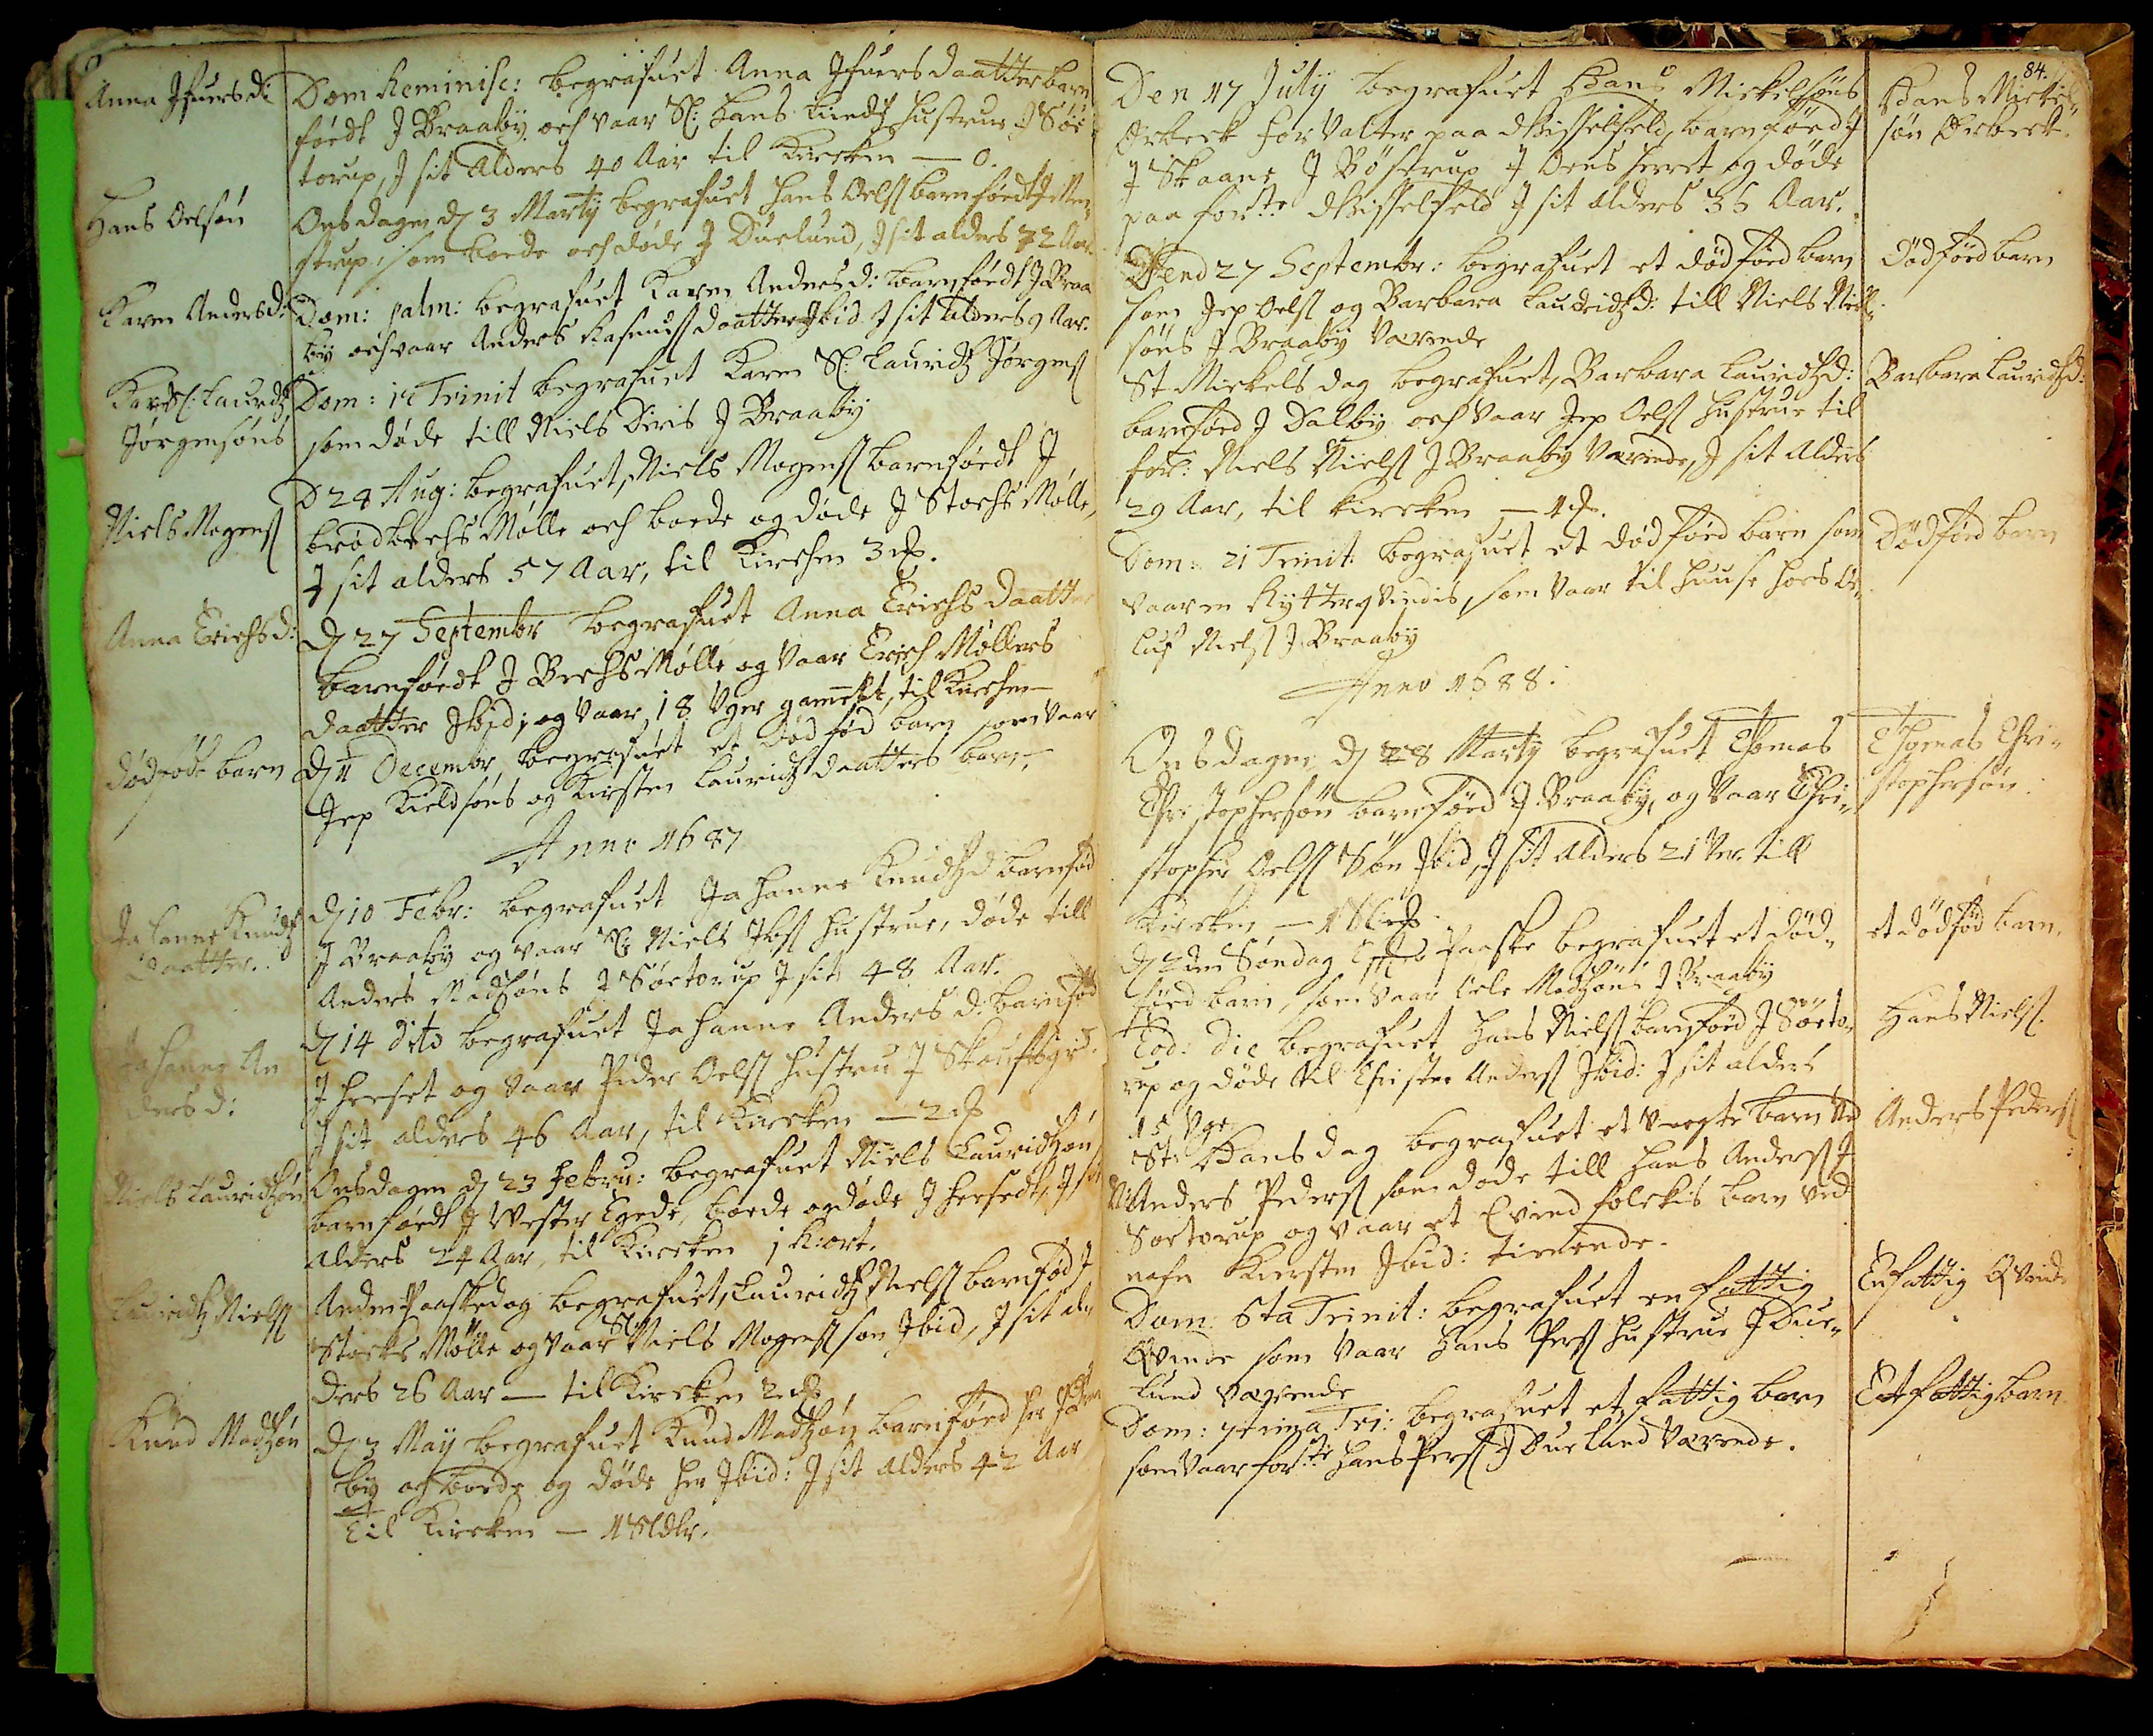Anna Jfuersd:
Dom Reminise: begrafuet Anna Jfuersdaatter barn
 føedt J Braaby och Vaar Sl: Hans Knuds## Hustrue J Søe
torup, J sit Alders 40 Aar til Kircken -0.
Hans Oelsøn
¬Onsdagen d 3. Marty begrafuet Hans Oels barnføedt J Men¬
strup, som boede och døde J Duelund, J sit alders 72 Aar.
Karen Andersd:
Dom: Palm: begrafuet Karen AndersD: barnføedt J Braa
by och vaar Anders Rasmus daatter Jbid, J sit alders 69 Aar.
Karen SL Lauridz##
Jørgensøns
Dom: 14 Trinit begrafuet Karen Sl: Lauridz Jørgens
som døde till Niels Diris J Braaby
Niels Mogens
D. 28 Aug: begrafuet, Niels Mogens barnføedt J
Brødbechs Mølle och boede og døde J Stochs Mølle,
J sit alders 57 Aar, til Kirchen 3 Mk.
Anna Erichsd:
D. 27 Septembr begrafuet Anna Erichsdaatter
barnføedt J Bechs Mølle og Vaar Erich Møllers
daatter Jbid; og Vaar 18 Uger gammelt, til Kirchen -
Dødfødt Barn 
D. 1 Decembr; begrafuet et dødfød barn som Vaar
Jep Kieldsøns og Kirsten Lauridzdaatters barn -
Anno 1687
Johanne Knuds
daatter 
D. 10 Febr: begrafuet Johanne Knudsd. barnføedt
J Braaby og Vaar Sl : Niels Jbs Hustrue, døde till
Anders Madsøns J Søetorup J sit 48 Aar.
Johanne An
dersd:. 
D. 14. Dito begrafuet Johanne Andersd. barnføedt
J Herset og vaar Peder Oels hustrue J Skoufsgrd##,
J sit alders 46 Aar, til Kircken - 2 Mk
Niels Lauridzøn
Onsdagen d 23 Febru: begrafuet Niels Lauridzøn
barnføedt J Vester Egede, boede och døde J Hersedt, J sit
alders 24 Aar, til Kircken 1 R: ort##
Lauridz Niels
Anden Paaskedag begrafuet Lauridz Niels, barnføed J
Stocks Mølle og Vaar SL Niels Mogens  Søn Jbid, J sit al¬
ders 26 Aar - til Kircken 2 Mk.##
Knud Madzøn
D. 3 May begrafuet Knud Madzøn, barnføed her J Braa
by## og boede og døde her Jbid: J sit alders 42 Aar.
Til Kircken - 1 Sl. dlr.
Dend 17 July begrafuet Hans Mickelsøns
Gebert## forvalter paa Gisselfeld, barnføed J
J Skaane J Bøstrup i Ornsherret og døde
paa forte Gisselfeld J sit alders 36 Aar.
84.
Hans Mickel
søn Gebert##
Dend 27 Septembr: begrafuet et dødføed Barn
som Jep Oels og Barbara Lauridzd: till Niels Niel¬
søns J Braaby Værende. 
Dødføed barn
Sk. Mickels dag begrafuet, Barbara Lauridsd:
barnføed J Dalby och Vaar Jep Oels hustrue til
fort## Niels Niels J Braaby Værende, J sit aldris
29 Aar, til Kircken - 1 Mk.
Barbara Lauridsd:
Dom: 21 Trinit. begrafuet et dødføed barn som
vaar en Rytterqvindis som Vaar til huuse hoes Oe¬
luf Niels J Braaby.
Dødføed barn
Anno 1688:
Onsdagen d 28 Marty begrafuet Thomas
Christophersøn barnføed J Braaby, og Vaar Chri¬
stopher Oels Søn Jbid, J sit Alders 21 Uer##, till
Kircken - 1 Sl Mark. 
Thomas Chri¬
stophersøn
D. 2dn Søndag Efter Paaske begrafuet et død
føed barn, som Vaar Oele Madz J Braaby.
Et dødfød barn
Eod: die begrafuet Hans Niels barnføed J Søeto¬
rup og døde til Christen Anders Jbid: J sit aldris
15 Uge. 
Hans Niels
St Hans dag begrafuet et U cgte Barn ##
## Anders Peders, som døde till Hans Anders J
Søetorup og Vaar et Qvindfolkis barn ved
Nafn Kirsten Jbid: tienende.
Anders Peders
Dom 6ta Trinit: begrafuet en fattig
Qvinde som Vaar Hans Pers hustrue, J Due¬
lund Værende.
En fattig Qvinde
Dom: 7tima Tri: begrafuet et fattig barn
som vaar forte## Hans Pers J Duelund Værende.
Eet fattig barn
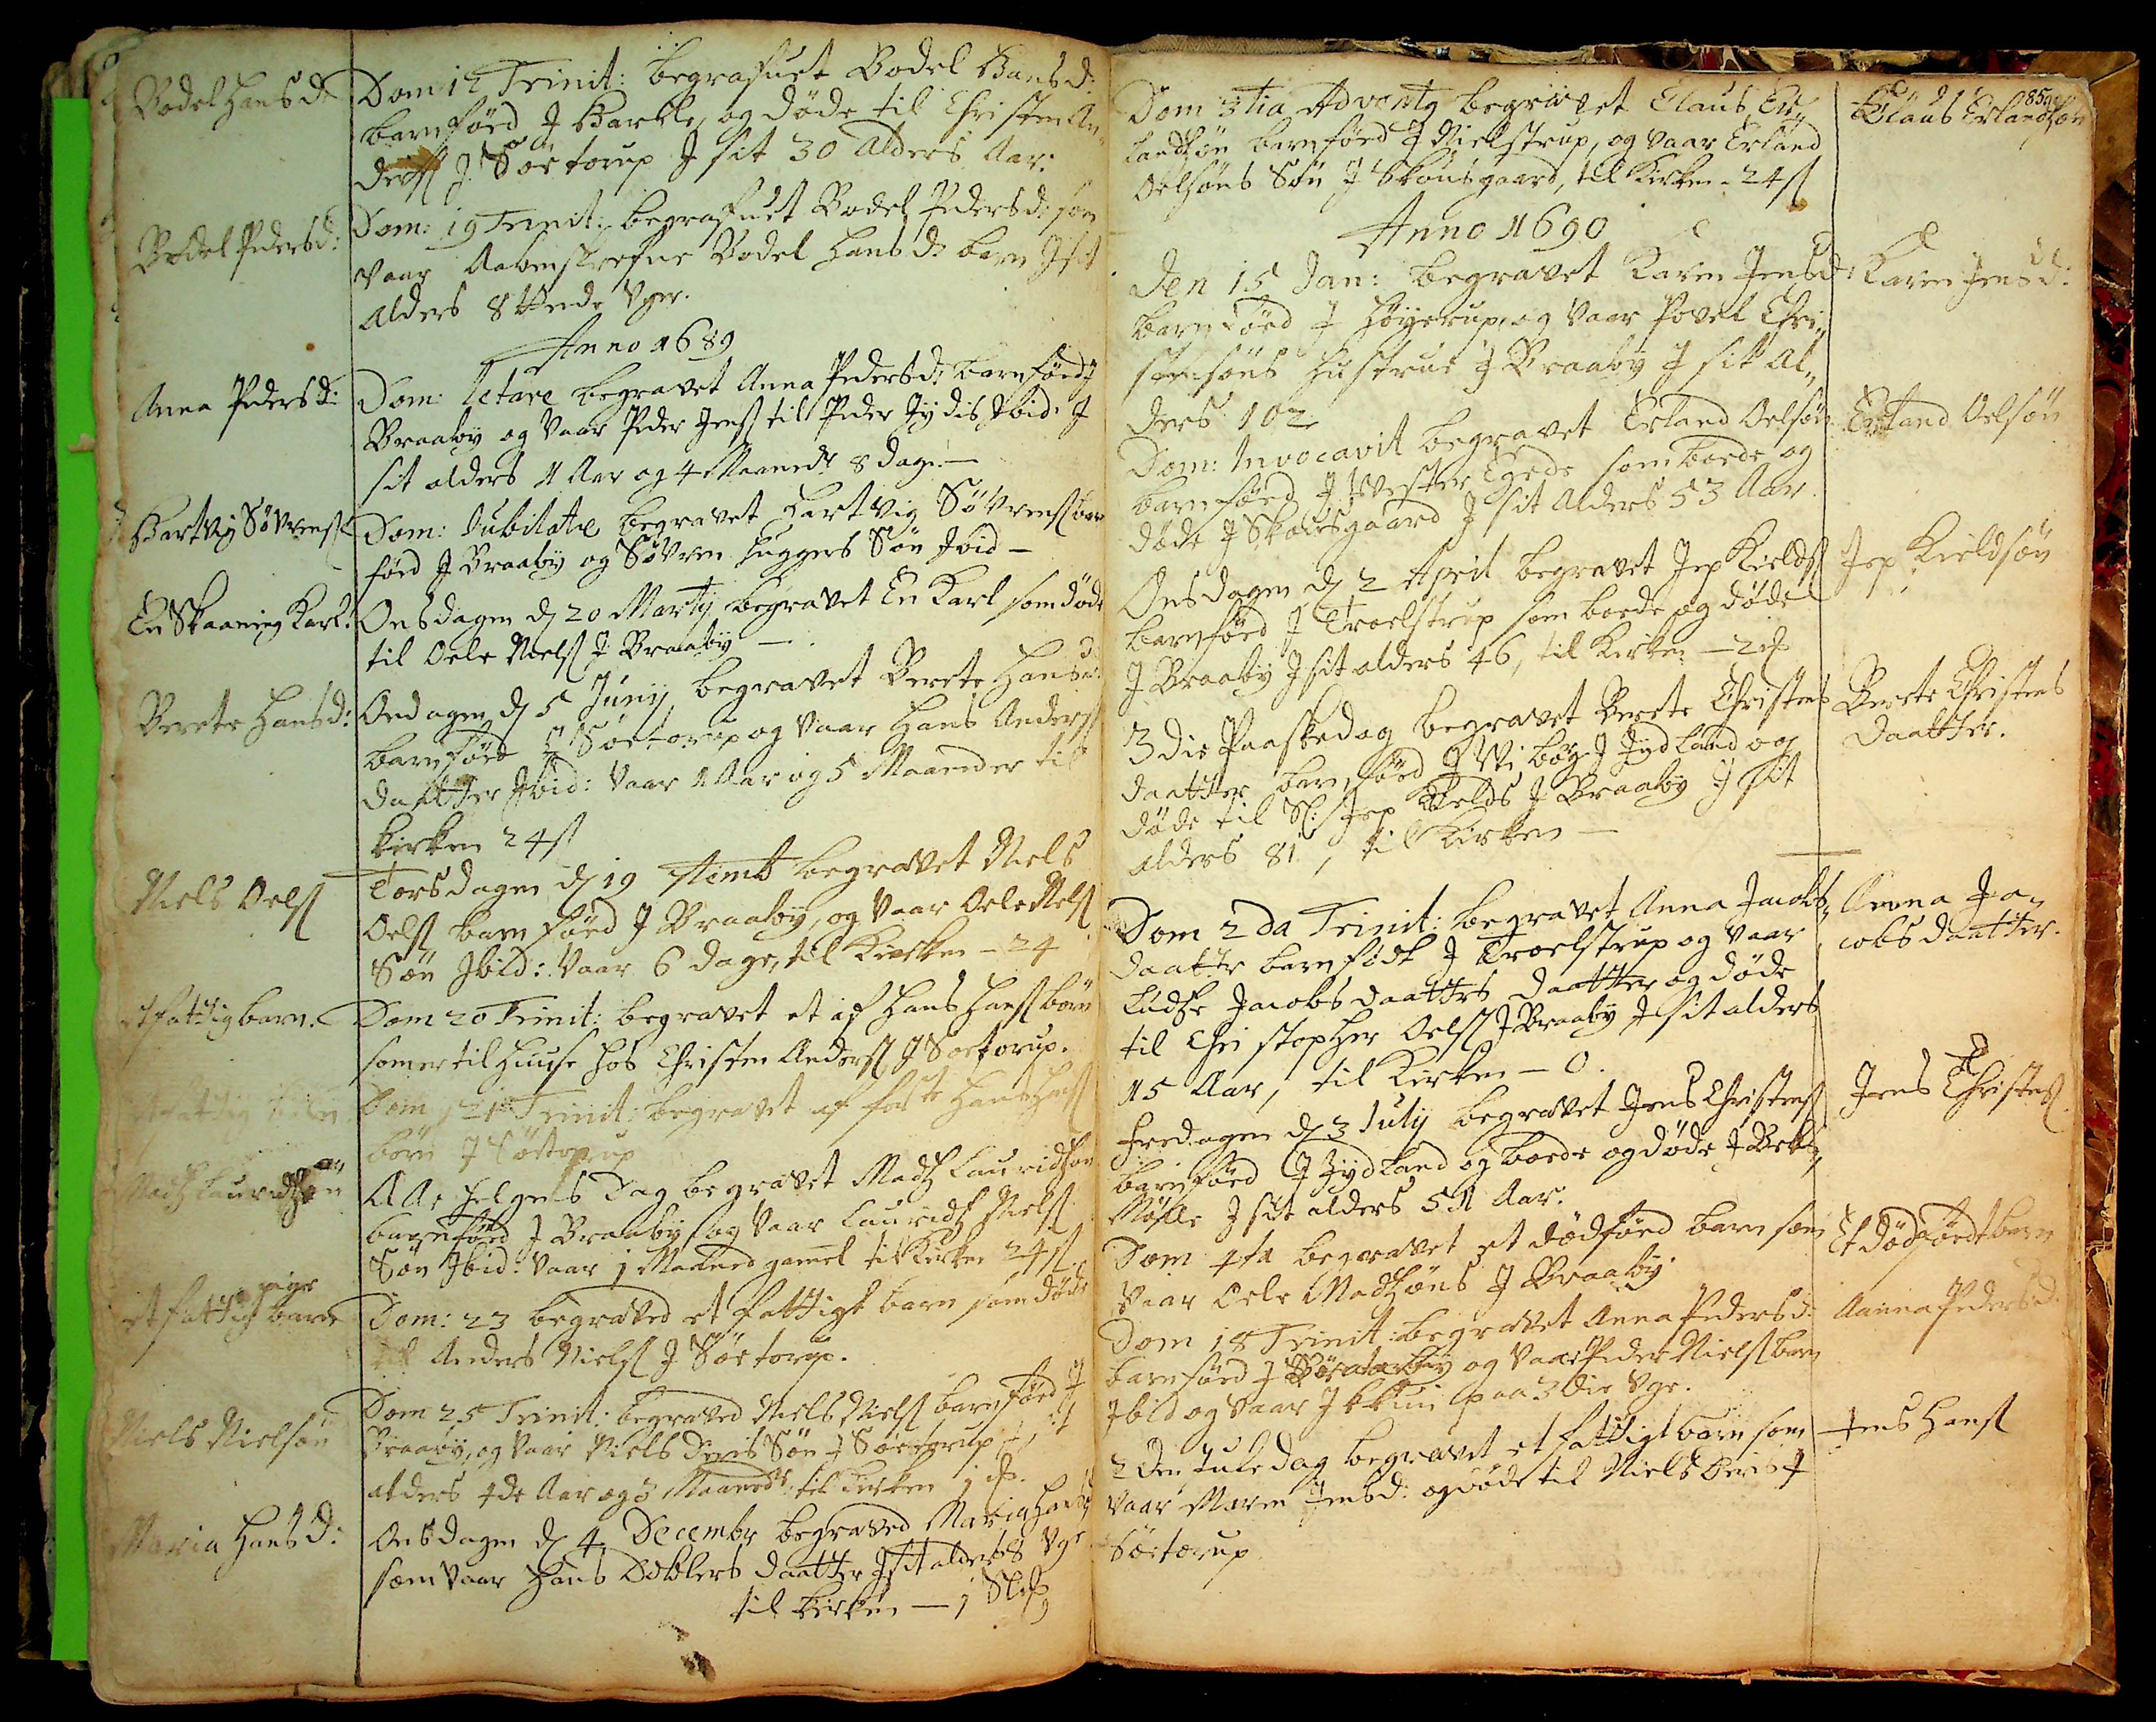Bodil Hansd: 
Dom: 12 Trinit: begrafuet Bodil Hansd:
barnføed J Harsle, og døde til Christen An¬
dersøn J Søetorup i sit 30 Alders Aar.
Bodel Pedersd:
Dom: 19 Trinit: Begrafuet Bodel Pedersd: som
Vaar Aabmskrifne Bodel Hansd: Barn, J sit
alders 8ttende## Uger.
Anno 1689
Anna Pedersd:
Dom: Letare begravet Anna Pedersd: barnføed J
Braaby og vaar Peder Jens til Peder Jydis Jbid, J
sit alders 1 Aar og 4 Maaned 8 Dage -
Hartvig Søvrensøn
Dom: Jubilate begravet Hartvig Søvrensøn barn
føed J Braaby og Søvren Huggers Søn Jbid -
En Skaaning Karl
Onsdagen d. 20 Marty begravet En Karl som døde
til Oele Niels J Braaby. -
Berete Hansd: 
Onsdagen d 5 Juny begravet Berete Hansd.
barnføed J Søetorup og Vaar Hans Anders
daatter Jbid: Vaar 1 Aar og 5 Maaneder, til
Kircken 24 Sk. 
Niels Oels
Torsdagen d. 19 7temb: begravet Niels
Oels, barnføed J Braaby, og Vaar Oele Niels
Søn Jbid: vaar 6 Dage, til Kircken -24 S.##
Et fattig barn
Dom 20 Trinit: begravet et af Hans Hans børn
som ere til Huuse hos Christen Anders J Søetorup.
Et fattig barn
Dom 21 Trinit: begravet et af forte Hans Hans
børn J Søetorup.
Madz Lauridzøn
Alle Helgenis Dag begravet Mads Lauridsøn
barnføed J Braaby og Vaar Lauridz Niels
Søn Jbid: Vaar 1 Maaned gammel til Kircken 24 Sk.
Et fattigt barn
##
Dom 23 begraved et fattigt barn som døde
til Anders Niels J Søetorup.
Niels Nielsøn
Dom 25 Trinit: begraved Niels Niels, barnføed J
Braaby, og Vaar Niels Diris Søn J Søetorup, J sit
alders 4de Aar og 3 Maaneder, til Kircken 1 Mk.
Maria Hansd:
Onsdagen d. 4 December begraved Maria Hansd
som Vaar Hans Doblers daatter J sit Alders 8 Uge,
til Kircken – 1 Slmk.
Dom 3tia Adventy begravet Claus Er¬
landzøn, barnføed J Nielstrup, og Vaar Erland
Oelsøns Søn J Skousgaard, til Kircken -24 Sk.
85.
Claus Erlandzøn
Anno 1690
Den 15 Jan: begravet Karin Jensd:
barnføed J Høyerup og Vaar Povel Chri¬
stensøns Hustrue J Braaby J sit al¬
ders 102. 
Karen Jensd:
Dom: Invocavit begravet Erland Oelsøn
barnføed J Wester Egede, som boede og
døde J Skousgaard J sit Alders 53 Aar
Erland Oelsøn
Onsdagen d. 2 April begravet Jep Kields
barnføed J Troelstrup, som boede og døde
J Braaby J sit alders 46, til Kirken -2 Mk.
Jep Kieldsøn
3die Paaskedag begravet Berete Christens
daatter, barnføed J Wiborg J Jydland og
døde til SL Jep Kields J Braaby J si
t alders 81, til Kirken - 
Berete Christens
datter.
Dom 2da Trinit: begravet Anna Jacobs¬
daatter barnfødt J Troelstrup og Vaar
Sidse Jacobsdaatters daatter og døde
til Christopher Oels J Braaby, J sit alders
15 Aar, til Kirken -0
Anna Ja¬
cobsdaatter 
Fredagen d 3.July begravet Jens Christens
barnføed J Jydland og boede og døde J Becks¬
Mølle J sit alders 51 Aar.
Jens Christens
Dom 4ta begravet et dødføed barn som
vaar Oele Madzøns J Braaby.
Et dødføedt barn
Dom 18 Trinit: begravet Anna Pedersd:
barnføed J Braaby/Sørtoerup## og Vaar Peder Niels barn
Jbid og Vaar Jkkun paa 3die Uge.
Anna## Pedersd:
2den Juledag begravet et fattigt barn som
var Maren Jensd: og døde til Niels Diris J 
Søetorup. 
Jens Hans